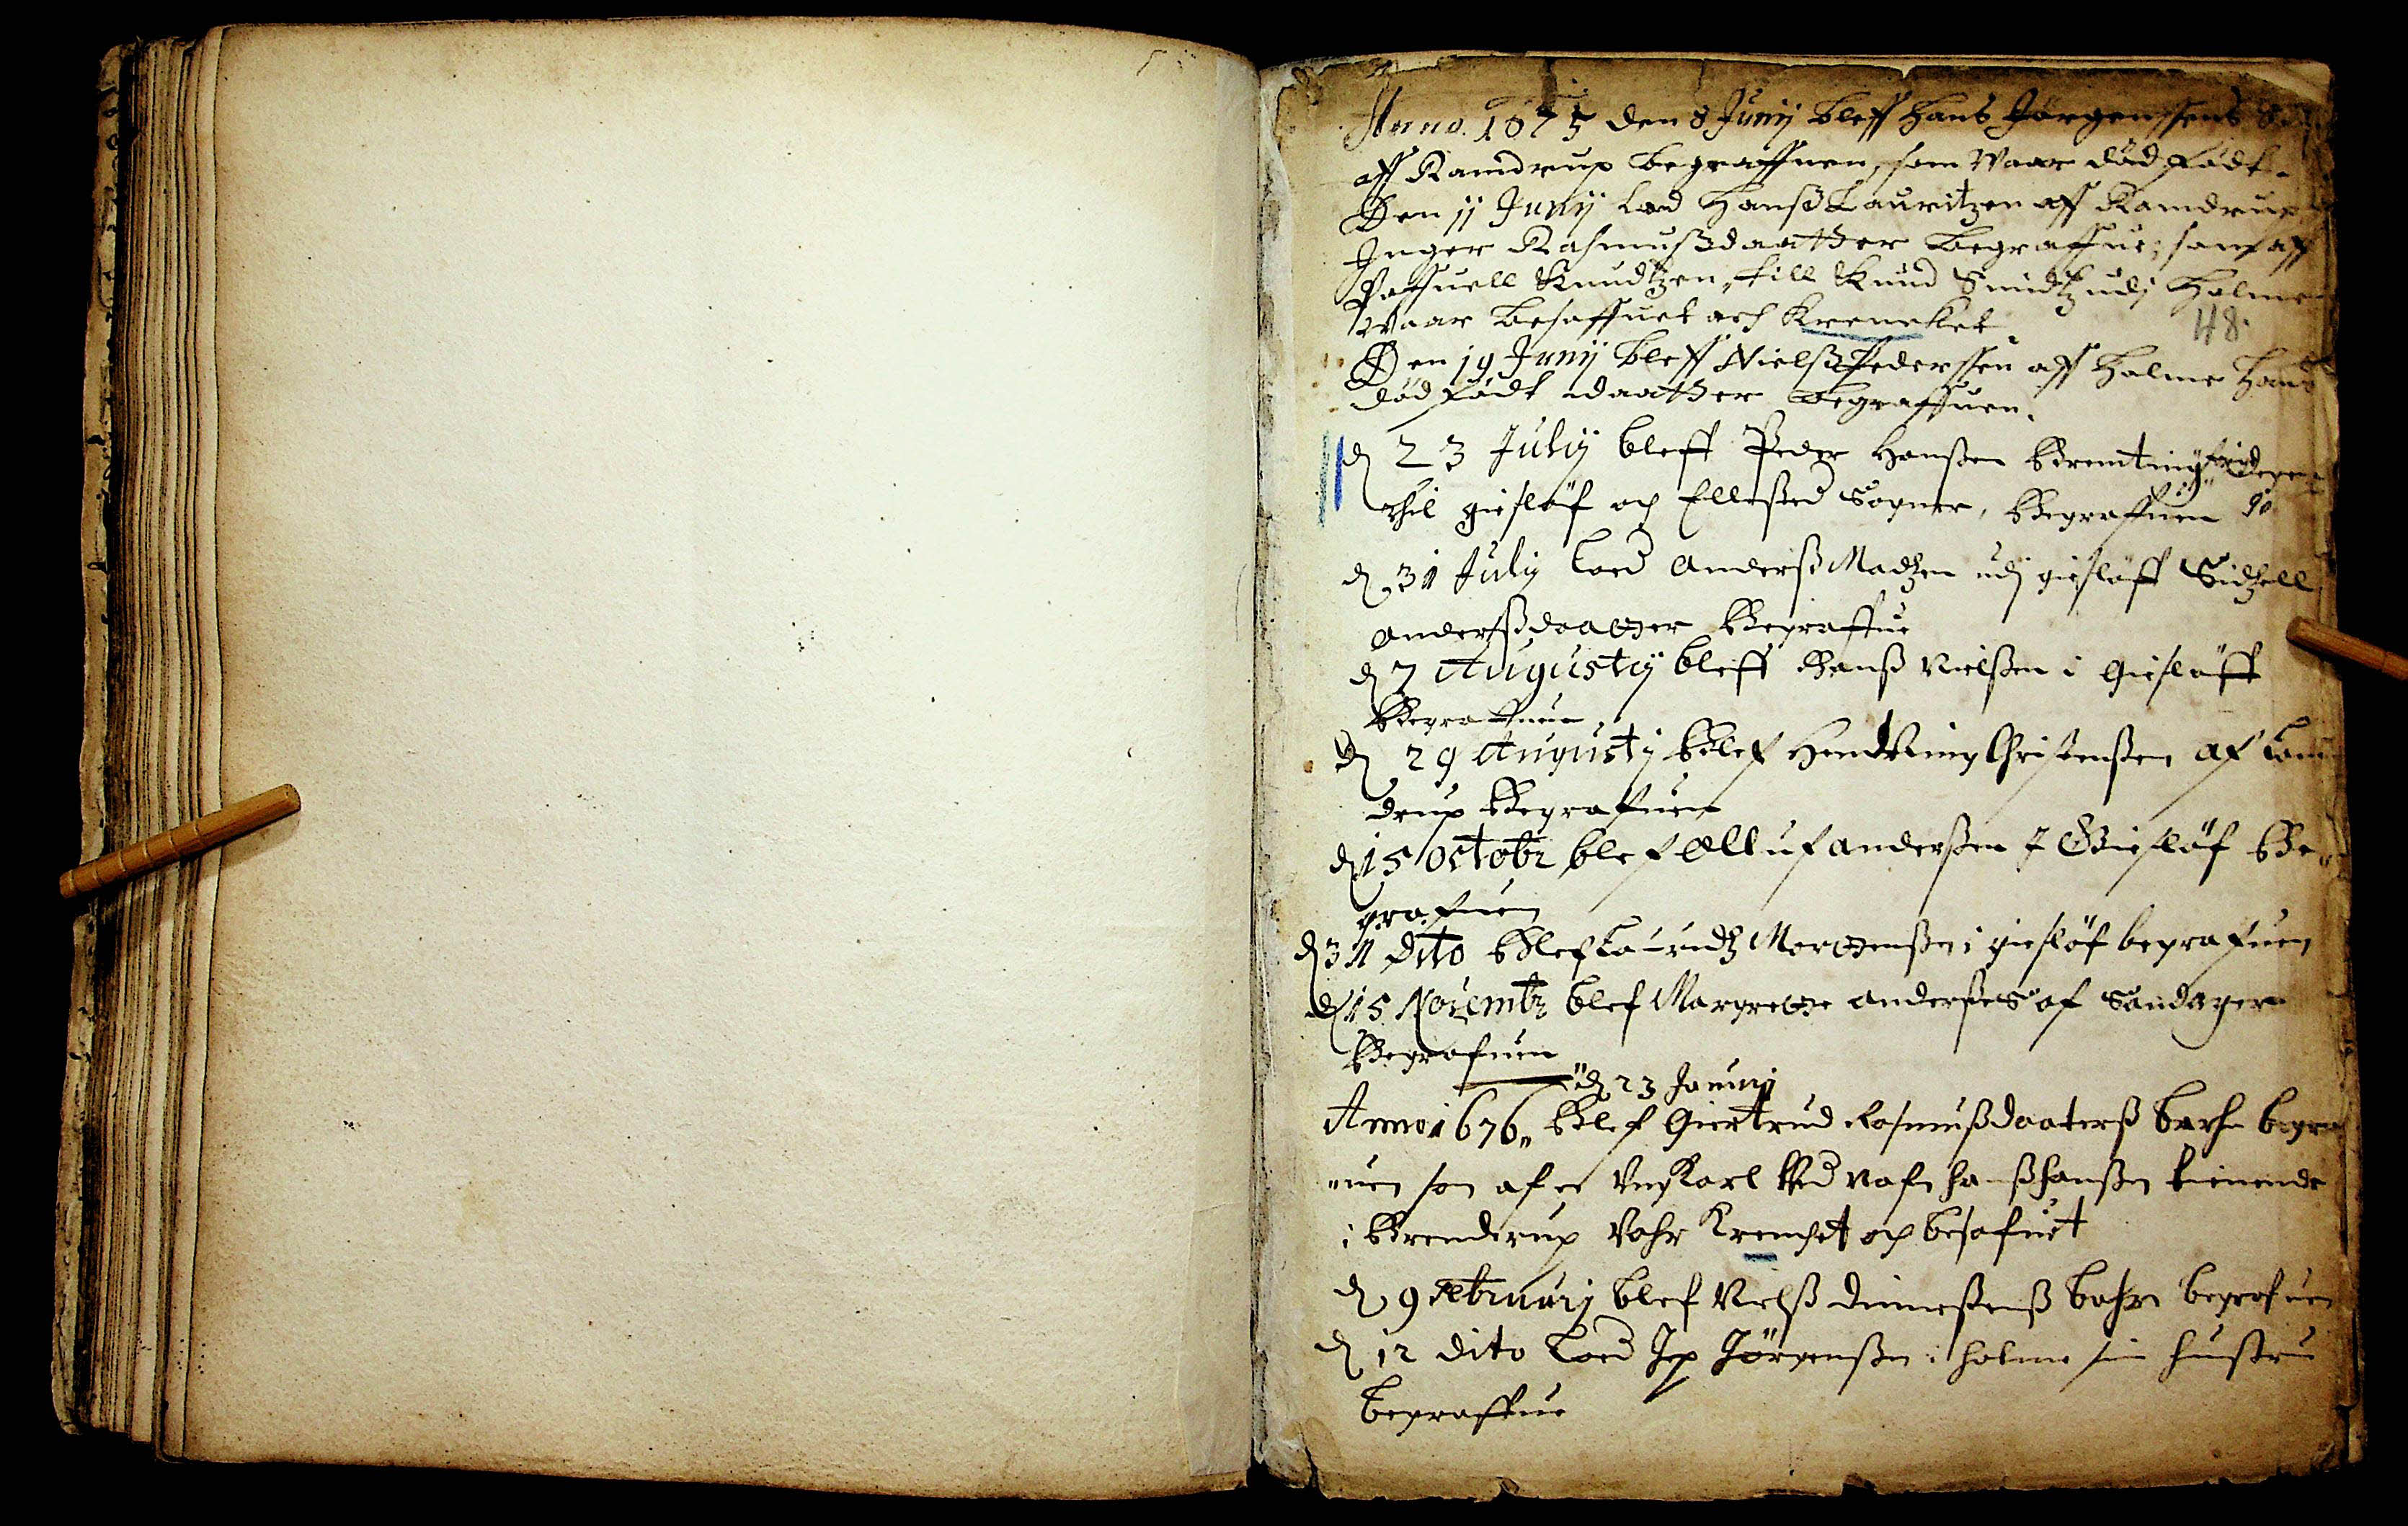48.
Anno 1675 den 8 Junij bleff Hans Jørgenssens Søn
aff Ramderup begraffuen, som vaar død født
Den 1 Junij Loed Hanss Lauritzen aff Ramdrup
Inger Rasmussdaatter begraffue som af
Poffuell Knudtzen, till Knud Smidtz udi Holme
vaar besuffuet och kreneket
Den 19 Junij bleff Niels Pederssen af Holme Hans
død født daatter begraffuen
forige
23 Julij bleff Peder Hanssen Bremting degen
thil Giesløf och Ellested Sogner, Begraffuen
D 31 Julij loed Anderss Madtzen udj giesløff Sidtzell
Anderssdaatter Begraffue
d 7 Adugustij bleff Hanss Nielssen i Giesløff
Begraffuen
d 29 Augustij blef Hem##ing Christenssen af Lam
drup begrafuen
d 15 Octobr blef Olluf Anderssen J Gireløf Be¬
grafuen
d 31 Dito Blef Lauridtz Morthenssen i giesløf begrafuen
d 15 Novembr blef Margrette Anderssens af Sandager
Begrafuen
" d 23 Janu##
Anno 1676 ,, Blef Giertrund Rasmussdaaterss Barhn begra
= uen som af en vng karl Ved Nafn Hanss Hanssen tienende
i Brenderup vahr krenchet oh besofuet
d. 9 Februarij blef Nielss Dinnessenss bahrn begrafuen
d 12 dito Loed Jep Jørgensen i holme sen hustru
begraffue
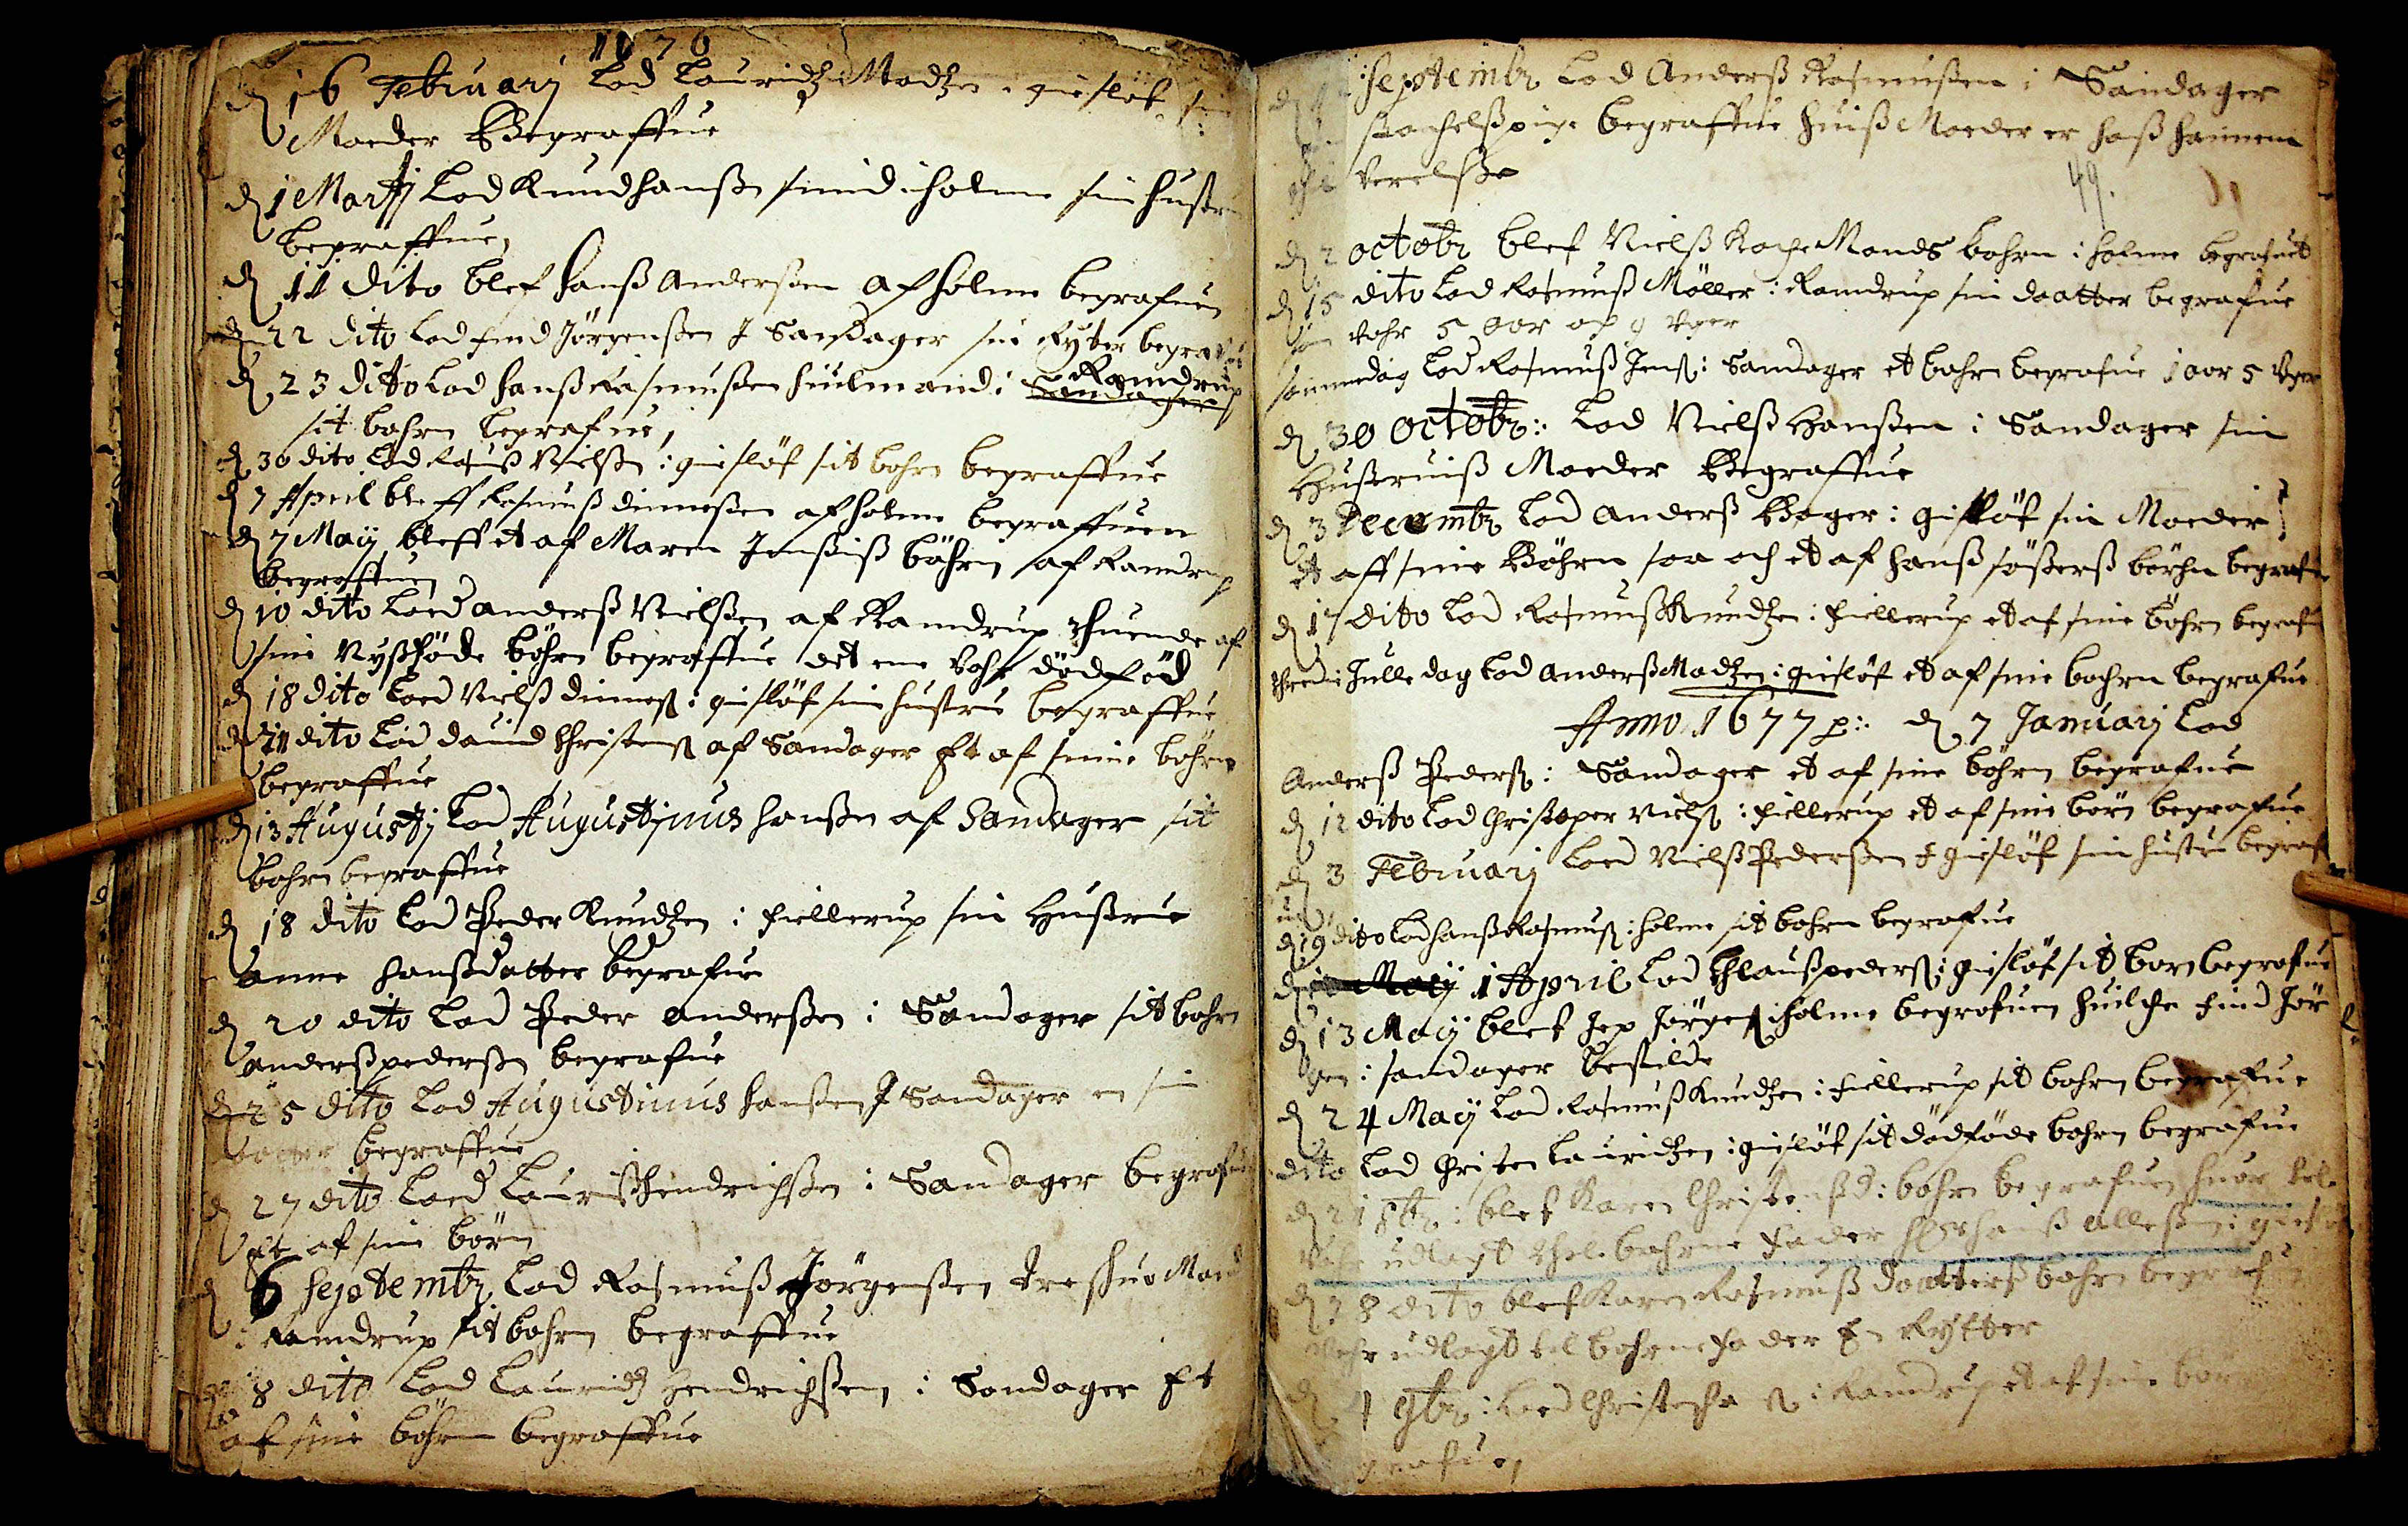1676
d 16 Februarij lod Lauridtz Madtzen i giesløf sin
Moeder Begraffue
d 1 Martsij Lod Knud hanssen smid i holme sin hustru
begraffue,
d 11 Dito blef Hanss Anderssen af holme begraffuen
Ded 22 Dito Lod find Jørgenssen i Sansdager sin Rytter
Ramdrup
d 23 dito lod Hanss Rasmussen hiulemand i Sandager
sit bahrn begrafue
d 30 dito lod Rasmuss Nielssen i giesløf sit bahrn begraffue
d 7 April bleff Rasmuss Dieressen af Holme begraffuen
d 7 Maij bleff et af Maren  Jenssiss bøhrn af Ramdrup
Begraffuen
D 18 dito Lod anderss Nielssen af Ramdrup tuende af
sine nyssføde bøhrn begraffue det ene vahre dødfød
d 18 dito loed Niels Dinness i giesløf sin hustrue begraffe
d 21dito Lod dauid Christens af Sandager Et af sinne bøhrn
begraffue
d 13 Augustij Lod Augustinus Hanssen af Sandager sit
bahrn begraffue
d 18 dito lod Peder Knudtzen i Fiellerup sin Hustrue
Anne Hansdatter begrafue
d 20 dito Lod Peder Anderssen i Sandager sit bohrn
Anderss Pedersen begrafue
d 5 Dito Lod Augustinus Hansen J Sandager en sine
datter begraffue
d 27 dito Loed Lauritss hendrichssen i Sandager begrafue
Et af sin børn
D 6 Septembr Lod Rasmuss Jørgensen Treskuo Mand
i Ramdrup sit bahrn begraffne
d 8 dito Lod Lauridtz Hendrichssen i Sandager Et
af sine bøhrn begraffue
49.
d ## Septembr Lod Anderss Rasmussen i Sandager
En stachelss pige begraffue huiss Moeder er hass ha##
## verelsse
d 2 Octobr blef Nielss Koche Mands bahrn i holme begrafuet
d 15. dito Lod Rasmuss Møller i Ramdrup sin daatter begrafune
som vahr 5 aar och 9 vger
sammedag lod Rasmuss Jens i Sandager et bahrn begrafue 1aar 5 vger
d 30 Octobr:. Lod Niels Hanssen i Søndager sin
Hustruis Moeder Begraffue
d 3 Decembr Lod Anderss Bager i gisløf sin Moeder
et aff sine Bøhrn s## och et af hans søsterss børhn begraffue
d 17 dito lod Rasmuss Knndtzen i Fiellerup et af sine bøhrn begraffue
thredie Julle dag tod Anders Madtzen i giesløf et af sine bohrn begraffue
Anno 1677 p: d 7 Januarij lod
Anderss Pederss i Søndager et af sine bøhrn begrafue
d 12 dito lod Christoper Niels i Fiellerup et af sine børn begraffue
d 3. Februarij loed Nielss Pederssen I giesløf sin hustru begraf
ue
d 19 dito lod hanss Rasmuss i holme sit bahrn begrafue
d 1 Maij 1 April lod Chlaus pederss i giesløf sit barn begraofue
d 13 Maij blef Jep Jørgens holme begrafuen huilche find Jør
gen i sandager bestilde
d 24 Maij Lod lRasmuss Knudtzen i Fiellerup sit bahrn begrafue
diti Lod Christen Lauridtzen i giesløf sit dødføde bohrn begraffue
d 21 8br: blef Karen Christenssd: bahrnsen begrafuen huor tel
vafr udlagt thie bahrne Fader ## Hanss ollessen i giesløf
d 28 dito blef Karen Rasmussdaatterss baøhrn begrafue
vahr udlagt tel bahrenefader EnJ Rytter
d 4 9br: lod Christen Hanss i Ramdrup et af sine børn
begrafue